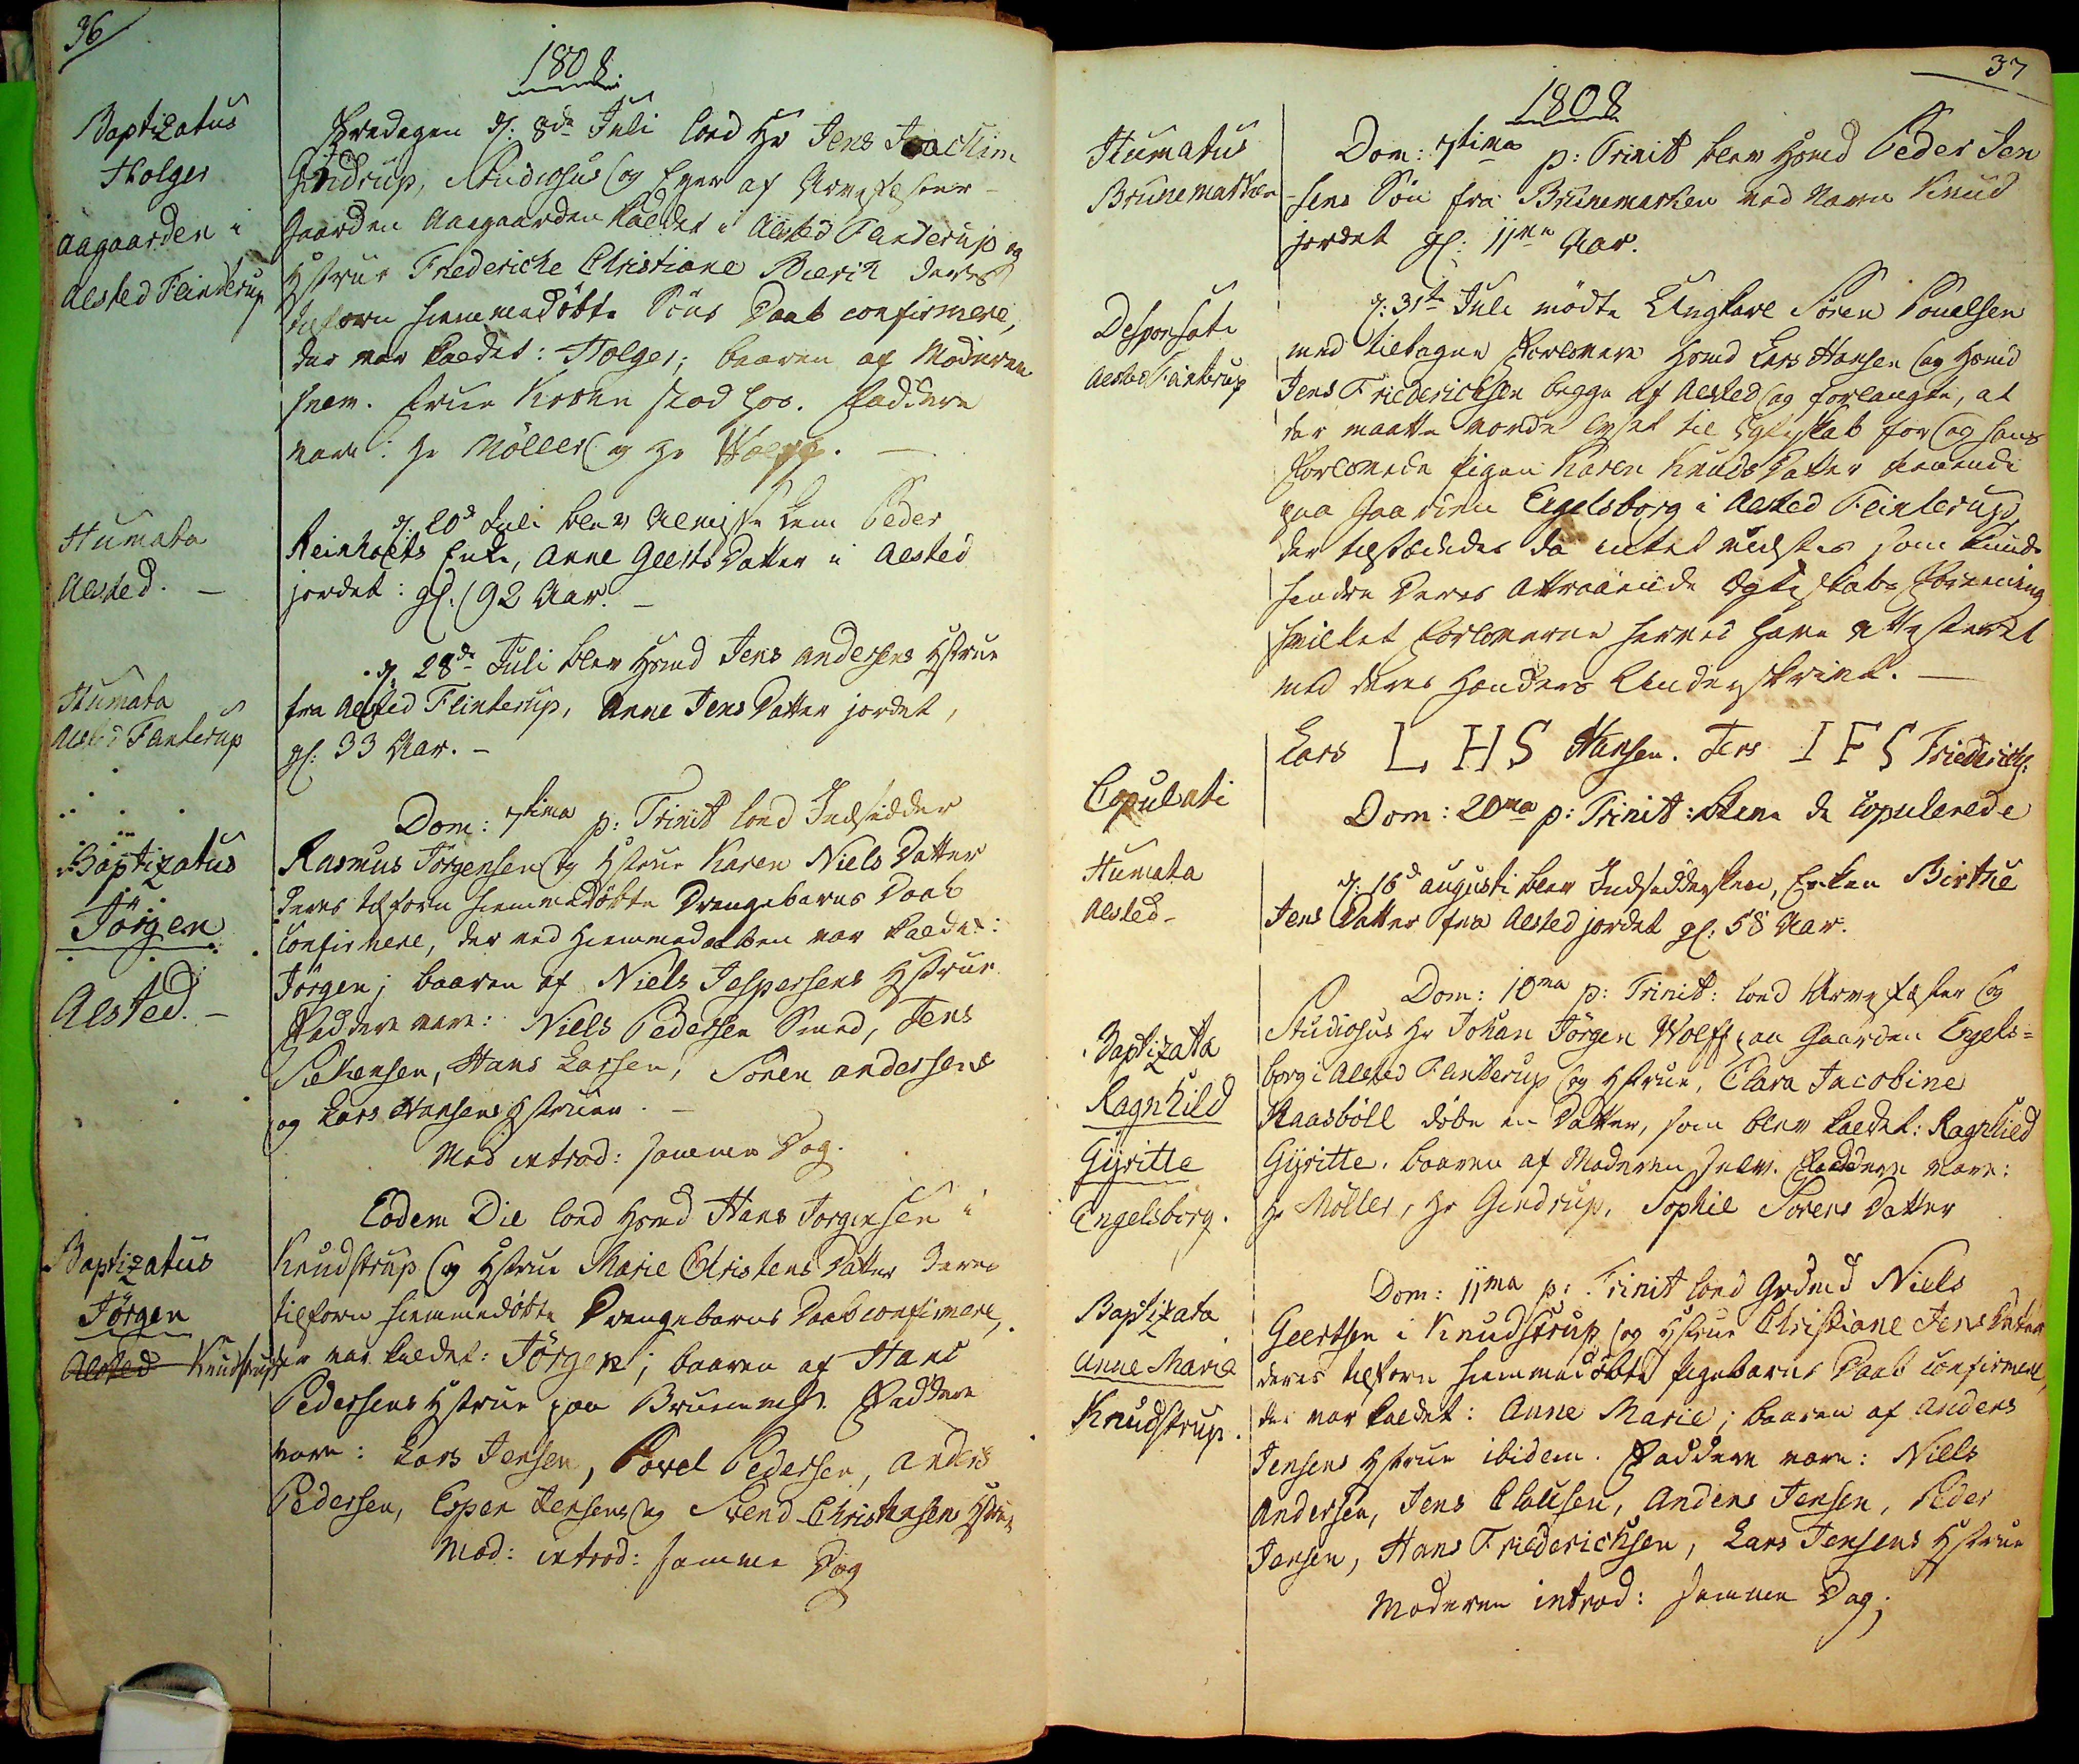36
Baptizatus
Holger
Aagaarden i
Alsted Flinterup
Humata
Alsted
Hunata
Alsted Flinterup
Baptizatus
Jørgen
Alsted.
Baptizatus
Jørgen
Alsted Knudstrup
1808
Fredagen d: 8de Juli loed Hr Jens Joachim
Gindrup, Studiosus og Eyer af Arvefæster
Gaarden Aagaarden kaldet i Alsted Flinterup og
Hstrue Frederiche Christiane Bierch deres
tilforn hiemmedøbte Søns Daab confirmere,
der var kaldet: Holger; baaren af Moderen
selv. Frue Krohn stod hos. Faddere
vare: Hr Møller og HrWolff.
d: 20de Juli blev Almisse dem Peder
Reinholts Enke, Anne Geerts Datter i Alsted
jordet gl: 92 Aar.
d: 28de Juli blev Hsmd Jens Andersens Hstrue
fra Alsted Flinterup, Anne Jens Datter jordet,
gl: 33 Aar.
Dom: 7tima p: Trinit loed Indsidder
Rasmus Jørgensen og Hstrue Karen Niels Datter
deres tilforn hiemmedøbte Drengebarns Daab
confirmere, der ved Hiemmedaaben var kaldet:
Jørgen; baaren af Niels Jespersens Hstrue.
Faddere vare: Niels Pedersen Smed, Jens
Silkensen, Hans Larsen, Søren Andersens
og Lars Hansens Hstrue.
Mod introd: samme Dag.
Eodem Die loed Hsmd Hans Jørgensen i
Knudstrup og Hstrue Marie Christens Datter deres
tilforn hiemmedøbte Drengebarns Daab confirmere,
der var kaldet: Jørgen, baaren af Hans
Pedersens Hustrue paa Brunem##. Faddere
vare: Lars Jensen, Povel Pedersen, Anders
Pedersen, Espen Jensens og Svend Christensens Hstruer
Mod: introd: samme Dag
Humatus
Brunemarken
Desponsati
Alsted Flinterup
Copulati
Humata
Alsted.
Baptizata
Ragnhild
Gyritte
Engelsborg.
Baptizata
Anne Marie
Knudstrup.
37
1808
Dom: 7tima p: Trinit blev Hsmd Peder Jen
sens Søn fra Brunemarken ved Navn Knud
jordet gl: 11ve Aar
d: 31te Juli mødte Ungkarl Søren Pouelsen
med tiltagne Forlovere Hsmd Lars Hansen og Hsmd
Jens Friderichsen begge af Alsted og forlangte, at
der maatte vorde lyset til Egteskab for og hans
forlovede Pigen Karen Knuds Datter boende
paa Gaarden Engelsborg i Asted Flinterup
der tilstædedes da intet vidstes, som kunde
hindre Deres Attraaende Ægteskabs Forning
hvilket Forloverne herved have attesteret
med deres Hænders Underskrivt.
Lars L H S Hansen. Jens I F S Friderichs:
Dom: 20ma p: Trinit: bleve de copulerede
d: 16de Augusti blev Indsiddersken Enken Birthe
Jens Datter fra Alsted jordet gl: 58 Aar.
Dom: 10ma p: Trinit: loed Arvefæster og
Studiosus Hr Johan Jørgen Wolff paa Gaarden Engels¬
borg i Alsted Flinterup og Hstrue, Clara Jacobine
Kaasbøll døbe een Datter, som blev kaldet: Ragnhild
Gyritte, baaren af Moderen selv. Faddere vare:
Hr Møller, Hr Gindrup, Sophie Sorens Datter
Dom: 11ma p: Trinit loed Grdmd Niels
Geertsen i Knudstrup og Hstrue Christiane Jens Datter
deres tilforn hiemmedøbte Pigebarns Daab confirmere
der var kaldet: Anne Marie; baaren af Anders
Jensens Hstrue ibidem. Faddere vare: Niels
Andersen, Jens Clausen, Anders Jensen, Peder
Jensen, Hans Friderichsen, Lars Jensens Hstrue
Moderen introd: samme Dag;
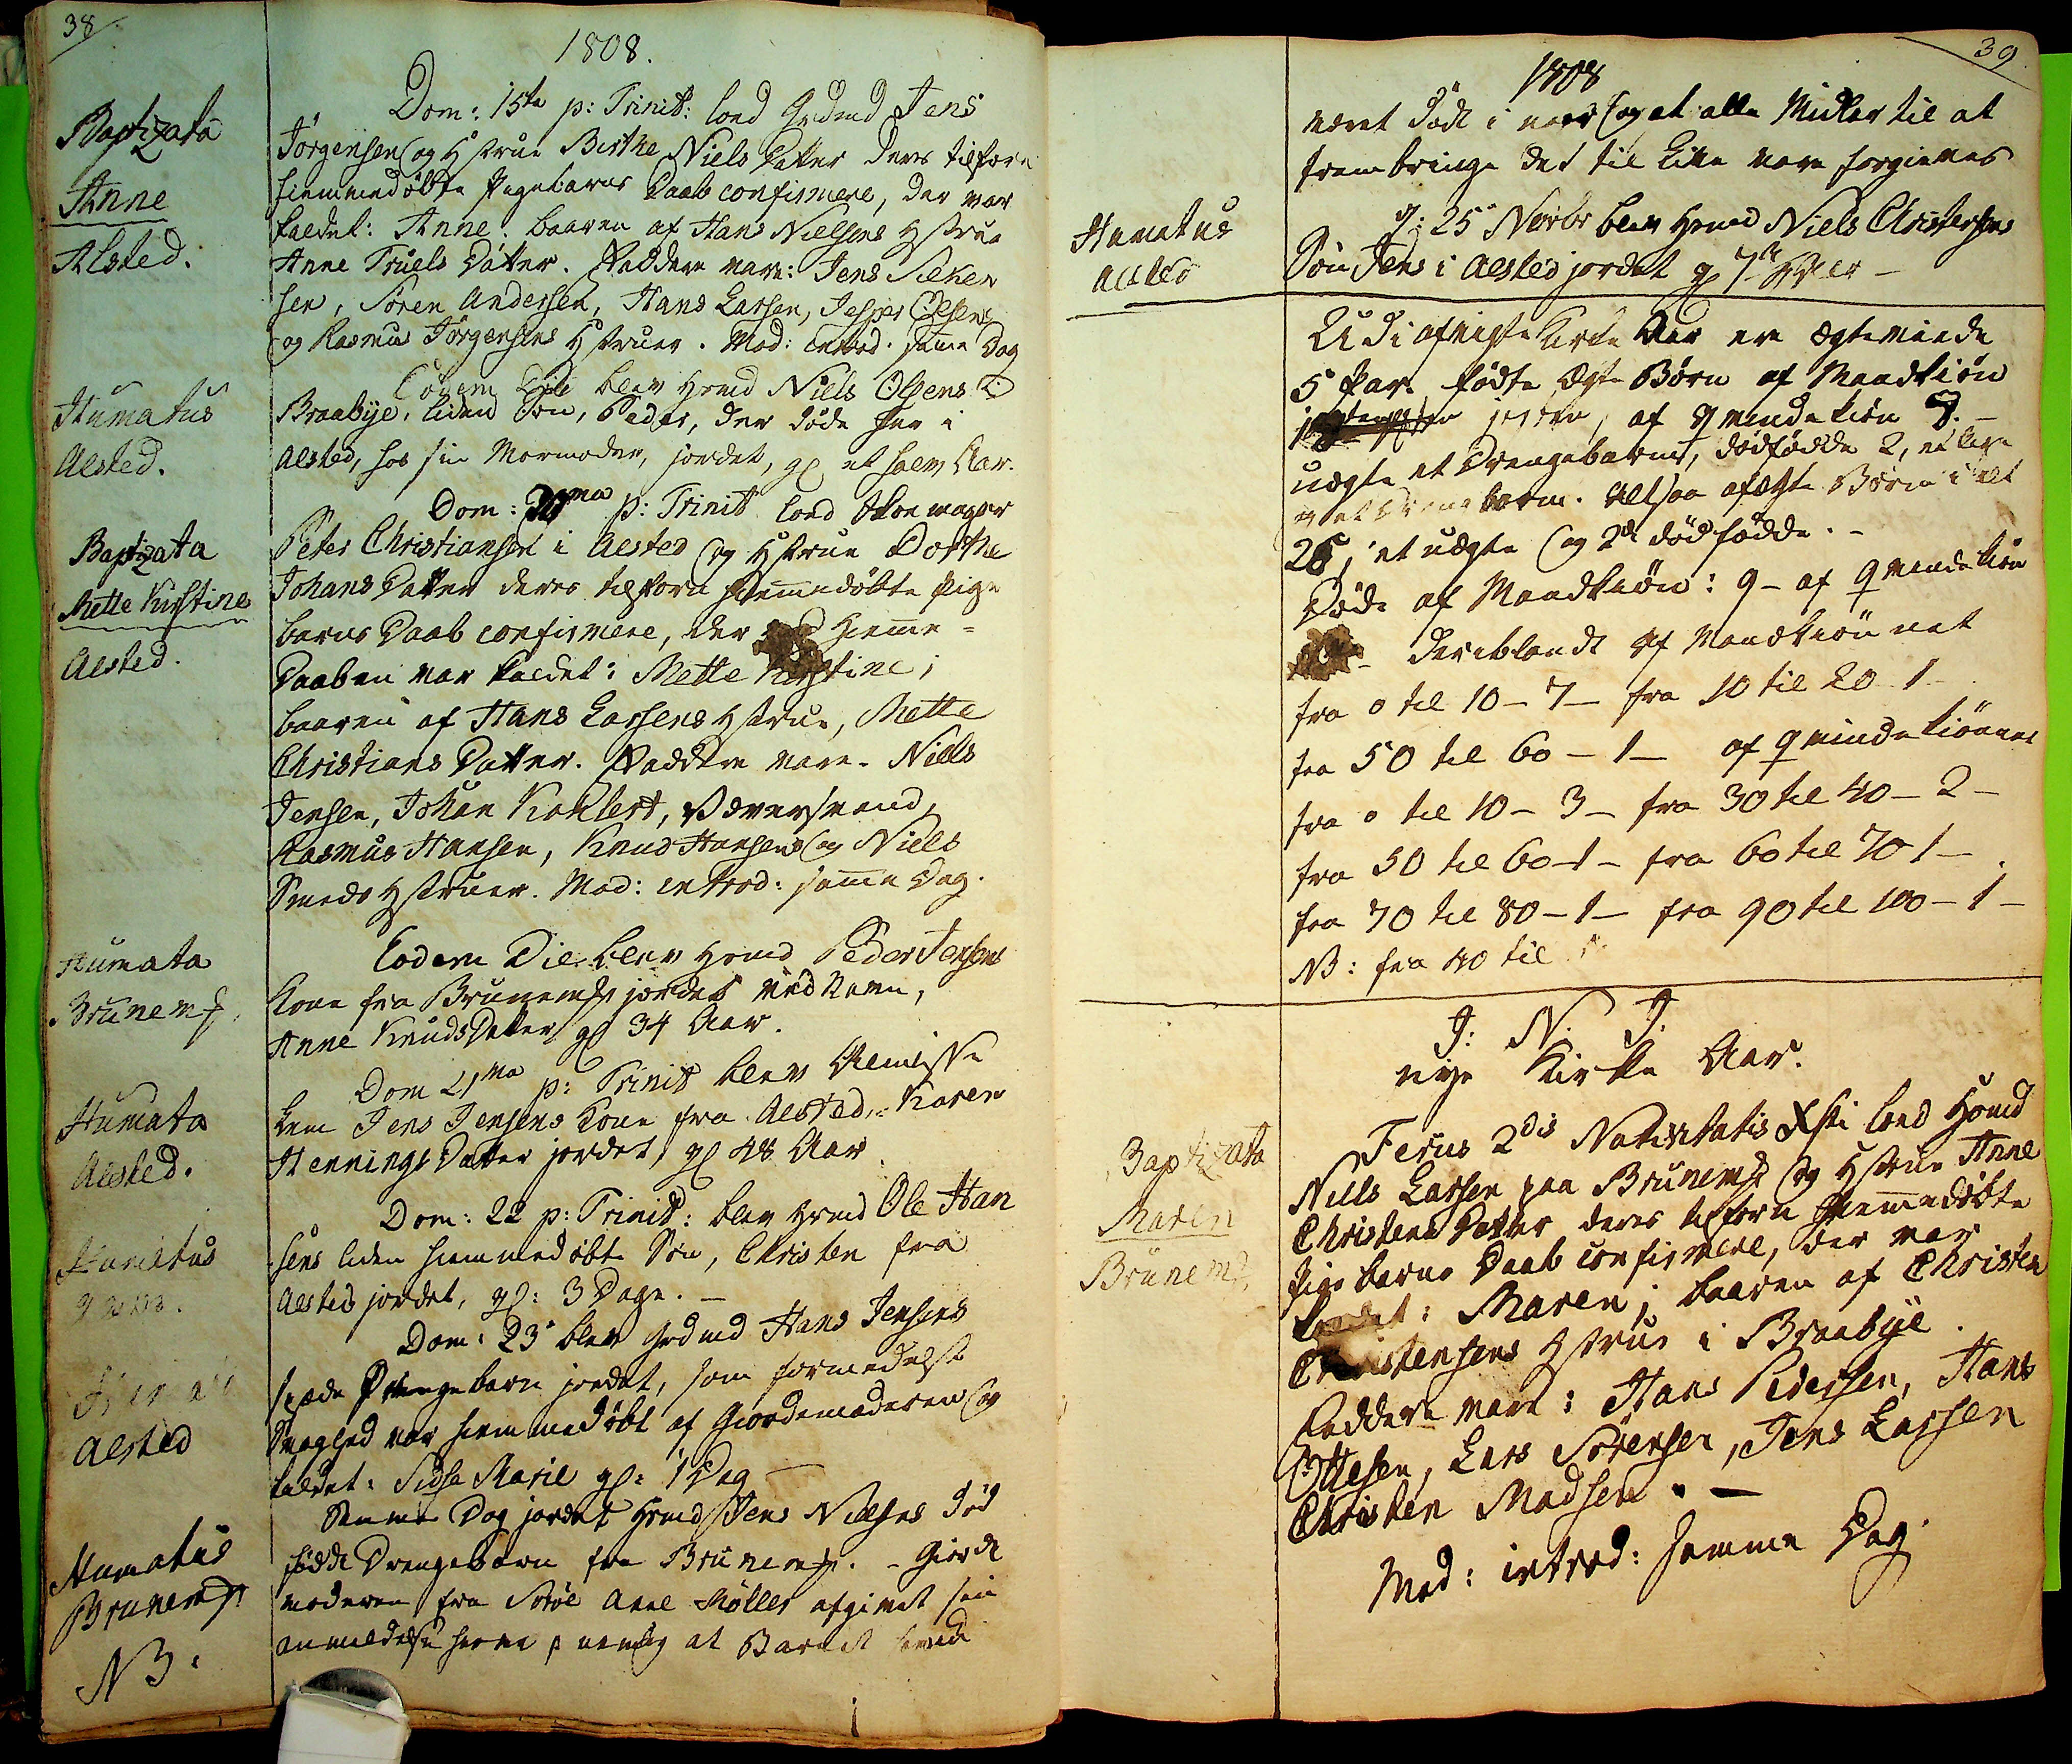38
Baptizata
Anne
Alsted.
Humatus
Alsted.
Baptizata
Mette Kirstine
Alsted.
Humata
Brunem##
Humata
Alsted.
Humatus
Alsted
Humatus
Alsted
Humatus
Brunem##
NB:
1808.
Dom: 15ta p: Trinit: loed Grdmd Jens
Jørgensen og Hstrue Birthe Niels Datter deres tilforn
hiemmedøbte Pigebarns Daab confirmere, der var
kaldet: Anne; baaren af Hans Nielsens Hstrue
Anne Truels Datter. Faddere vare: Jens Silken
sen, Søren Andersen, Hans Larsen, Jesper Olsens
og Rasmus Jørgensens Hstruer. Mod: introd: samme Dag
Eodem Die blev Hsmd Niels Olsens i
Braabye, liden Søn, Peder, der døde her i
Alsted, hos sin Mormoder jordet, gl at halv Aar.
Dom: 20ma p: Trinit. lod Skoemager
Peter Christiansen i Alsted og Hstrue Dorthe
Johans Datter deres tilforn hiemmedøbte Pige
barns Daab confirmere, der ved Hiemme¬
Daaben var kaldet: Mette Kirstine;
baaren af Hans Larsens Hstrue, Mette
Christians Datter. Faddere vare. Niels
Jensen, Johan Kohleret, Væversvend,
Rasmus Hansen, Knud Hansens og Niels
Smeds Hstruer. Mod: introd: samme Dag.
Eodem Die blev Hsmd Peder Jensens
Kone fra Brunem## jordet ved Navn,
Anne Knuds Datter gl 34 Aar.
Dom 21ma p: Trinit blev Almisse
Lem Jens Jensens Kone fra Alsted, Karen
Hennings Datter jordet gl 48 Aar
Dom: 22 p: Trinit: blev Hsmd Ole Han
sens liden hiemmedøbte Søn, Christen fra
Alsted jordet gl: 3 Dage.
Dom: 23de blev Grdmd Hans Jensens
spæde Drengebarn jordet, som formedelst
Svaghed var hiemmedøbt af Giordemoderen og
kaldet: Sidse Marie gl 1 Dag
Samme Dag jordet Hsmd Jens Nielsens død
fødte Drengebarn fra Brunem##. Gioede
moderen fra Sorøe Anne Møller afgivet sin
anmeldelse her## nemlig at Barnet f##
Hamatus
Alsted
Baptizata
Maren
Brunem##.
39
1808
været dødt i ## og at alle Midler til at
frembringe det til Live vare forgieves
d: 25de Novbr blev Hsmd Niels Christensens
Søn Jens i Alsted jordet gl 7## ##
Udi afvigte Kirke Aar ere Ægteviede
5 Par. fødte Ægte Børn af Mandskiøn
## 17, af Quindekiøn 8. -
uægte et Drengebarn dødfødde 2, et Pige
og et Dreng barn.  Altsaa afægte Børn i alt
25, et uægte og 2de dødfødde.
Døde af Mandkiøn: 9 - af Gvindekiøn
## deriblande af Mandkiønnet
fra 0 til 10 - 7 - fra 10 til 20 - 1.
fra 50 til 60 - 1-  af Qvindekiønnet
fra 0 til 10 - 3 - fra 30 Jul 40 - 2 -
fra 50 til 60 - 1 - fra 60 til 70 1 -
fra 70 til 80 - 1 - fra 90 til 100 - 1 -
NB: fra 40 til ##
J: N: J:
nye Kirke Aar.
Feriis 2dis Nativitatis Xsti loe Hsmd
Niels Larsen paa Brunm## og Hstrue Anne
Christens Datter deres tilforn hiemmedøbte
Pige barns Daab confirmere, der var
kaldet: Maren; baaren af Christen
Christensens Hstrue i Braabye
Faddere vare: Hans Pedersen, Hans
Ottesen, Lars Sørensen, Jens Larsen
Christen Madsen
Mod: introd: samme Dag